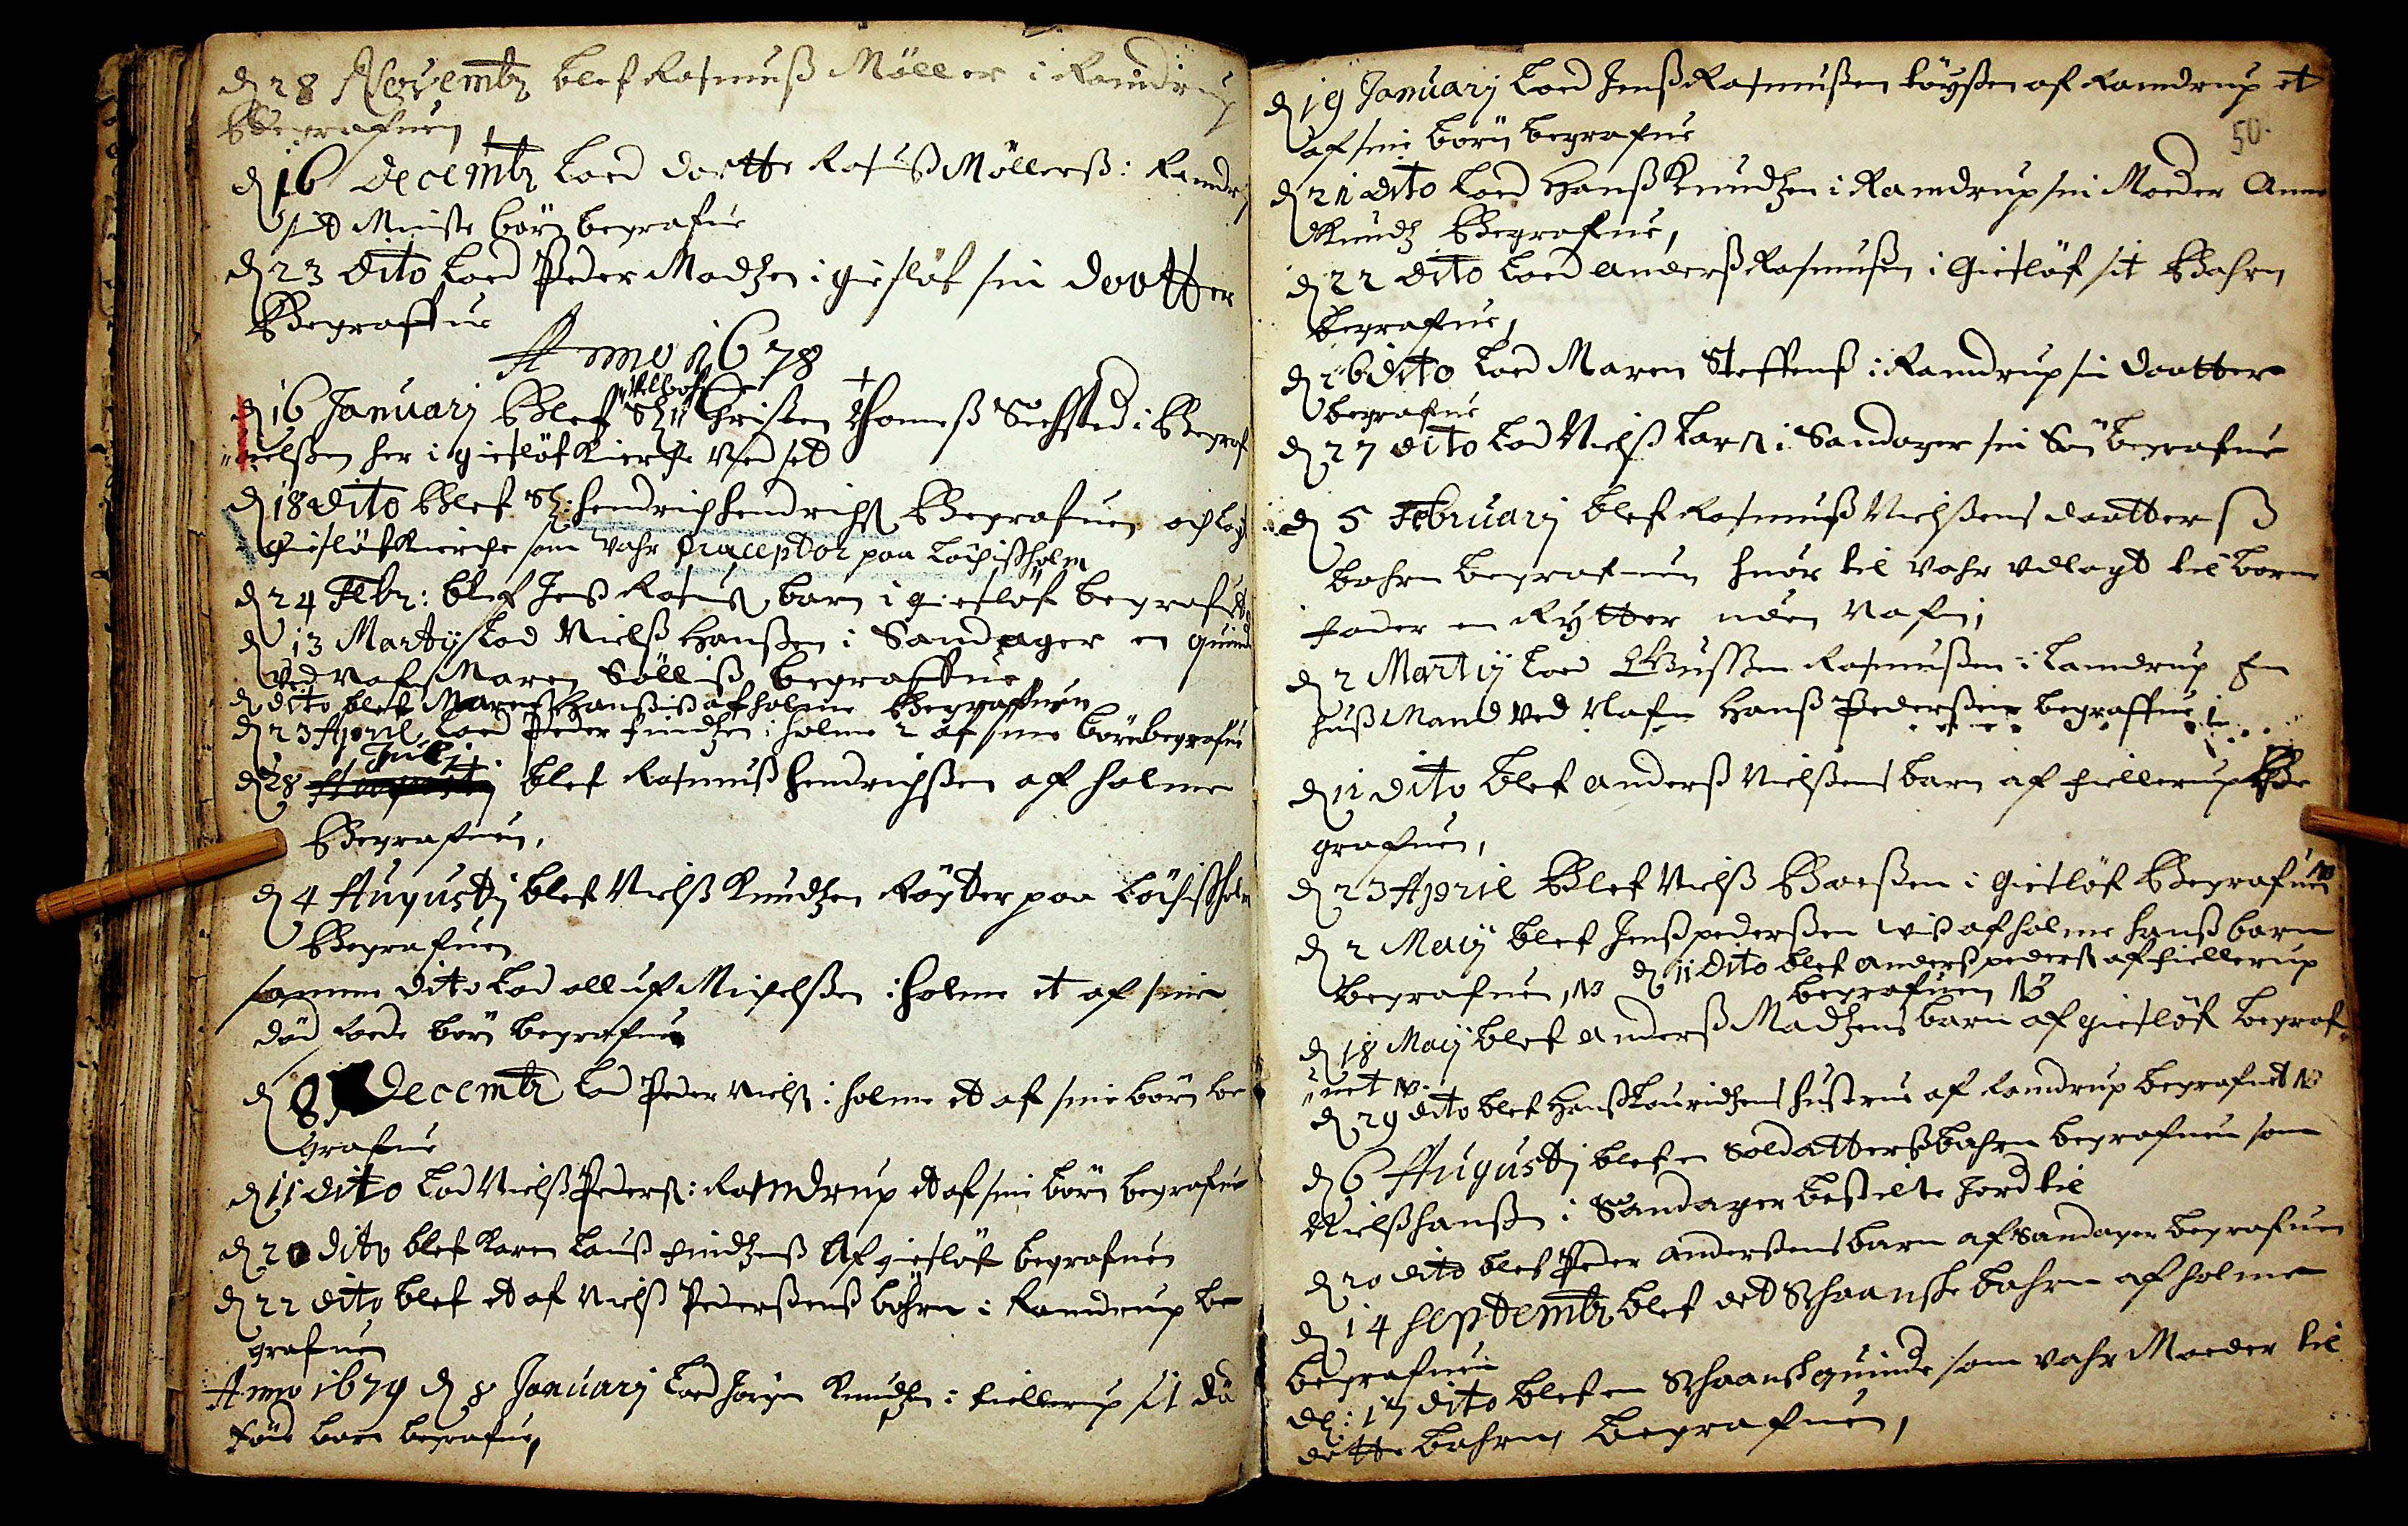d 28 Novembr blef Rasmuss Møller i Ramdrup
Begrafuen
d 16 Decembr loed dortte Rasmuss Møllers i Ramdrup
sit Minste børn begrafue
d 23 Dito Loed Peder Madtzen i giesløf sin daattter
Begraffue
Anno 1678
Velbohrne
d 16 Januarij Bleff ,, Christen Tomess Seehsted i Begraf
= uelssen her i gietsøf Kierche ned set
d 18 dito Bleff Sl: Hendrich Hendrichs Begrafuen och lagt
i Giesløf kierche som vahr Praceptor paa Løchisholm
d 24 Febr: blef Jens Rasmuss barn i giesløf begrafuet
d 13 Martij lod Nielss Hansen i Sandvger en quinde
ved nafn Maren Sølliss begraffue
d dito blef Maren Hanssis af holme begraffuen
d 23 April lod Peder Findtzen i holme 2 af sine børn begrafvue
Julj
d 8 Augustij blef Rasmuss Hendrichsen af holme
Begraffuen.
d 4 Augustij blef Nielss Knudtzen Røgter paa Løchisholm
Begrafuen
samme dito Lod olluff Michelssen i holme et af sine
død foede børn begraffue
d 8 Decembr lod Peder Nielss i holme et af sene born be
grafue
d 11 dito Lod Niels Peders i Ramdrup et af sine børn begrafue
d 20 dito blef Karen Lauss Fidztenss Af giesløf begrafuen
d 22 dito blef et af Niels Pederssens  bøhrn i Ramdrup be
grafnen
Anno 1679 d 8 Januarij lod Jørgen Knudtzen i Fiellerup sit død
føde barn begrafuen
50.
d 19 Januarij loed Jenss Rasmussen tøysten af Ramdrup et
af sine børn begrafue
d21 Dito loed Hanss Knudtzen i Ramdrup sin Moeder Anne
Knudtz begraffue,
d 22 Dito loed Anderss Rasmussen i Gietløf sit Bahrn
begraffue,
d 26 dito loed Maren Steffenss i Ramdrup sin daatter
begraffuet
d 27 dito lod Nielss Larss i Sandager sin Søn begrafue
d 5 Februarij blef Rasmuss Nielssens daatterss
bahrn begrafuen huor til vahr vdlagt til barne
fader en Rytter uden Nafn;
d 2 Martij loed Gustssen Rasmussen i Lamdrup en
Huss Mand ved Nafn Hanss Pederssen begraffue;
d 11 dito blef Anderss Nielssens barn af Fiellerup Be
grafuen,
d 23 Apriil Blef Nielss Boessen i Giesløf Begrafuen
d 2 Maij blef Jenss pederssen ##is af holme hHanss barn
begrafuen. NB d 11 Dito blef Anderss peders af Fiellerup
begrafuen NB
d 18 Maij blef Anderss Madtzens barn af giesløf begraf¬
= uet NB.
d 29 dito blef Hanss Louridtzens hustrue af Ramdrup begrafuet NB
d 6 Augustij blef en Soldatterss bahrn begrafuen som
Nielss Hansen i Sandager bestielte Jord til
d 20 dito blef Peder Andersens barn af Sandagen begrafuen
d. 14 Septembr blef det Schaanske bahrn af holme
begrafven
de: 17. dito bleff en Schaansk quinde som vafr Morder til
dette bahrn begrafuen
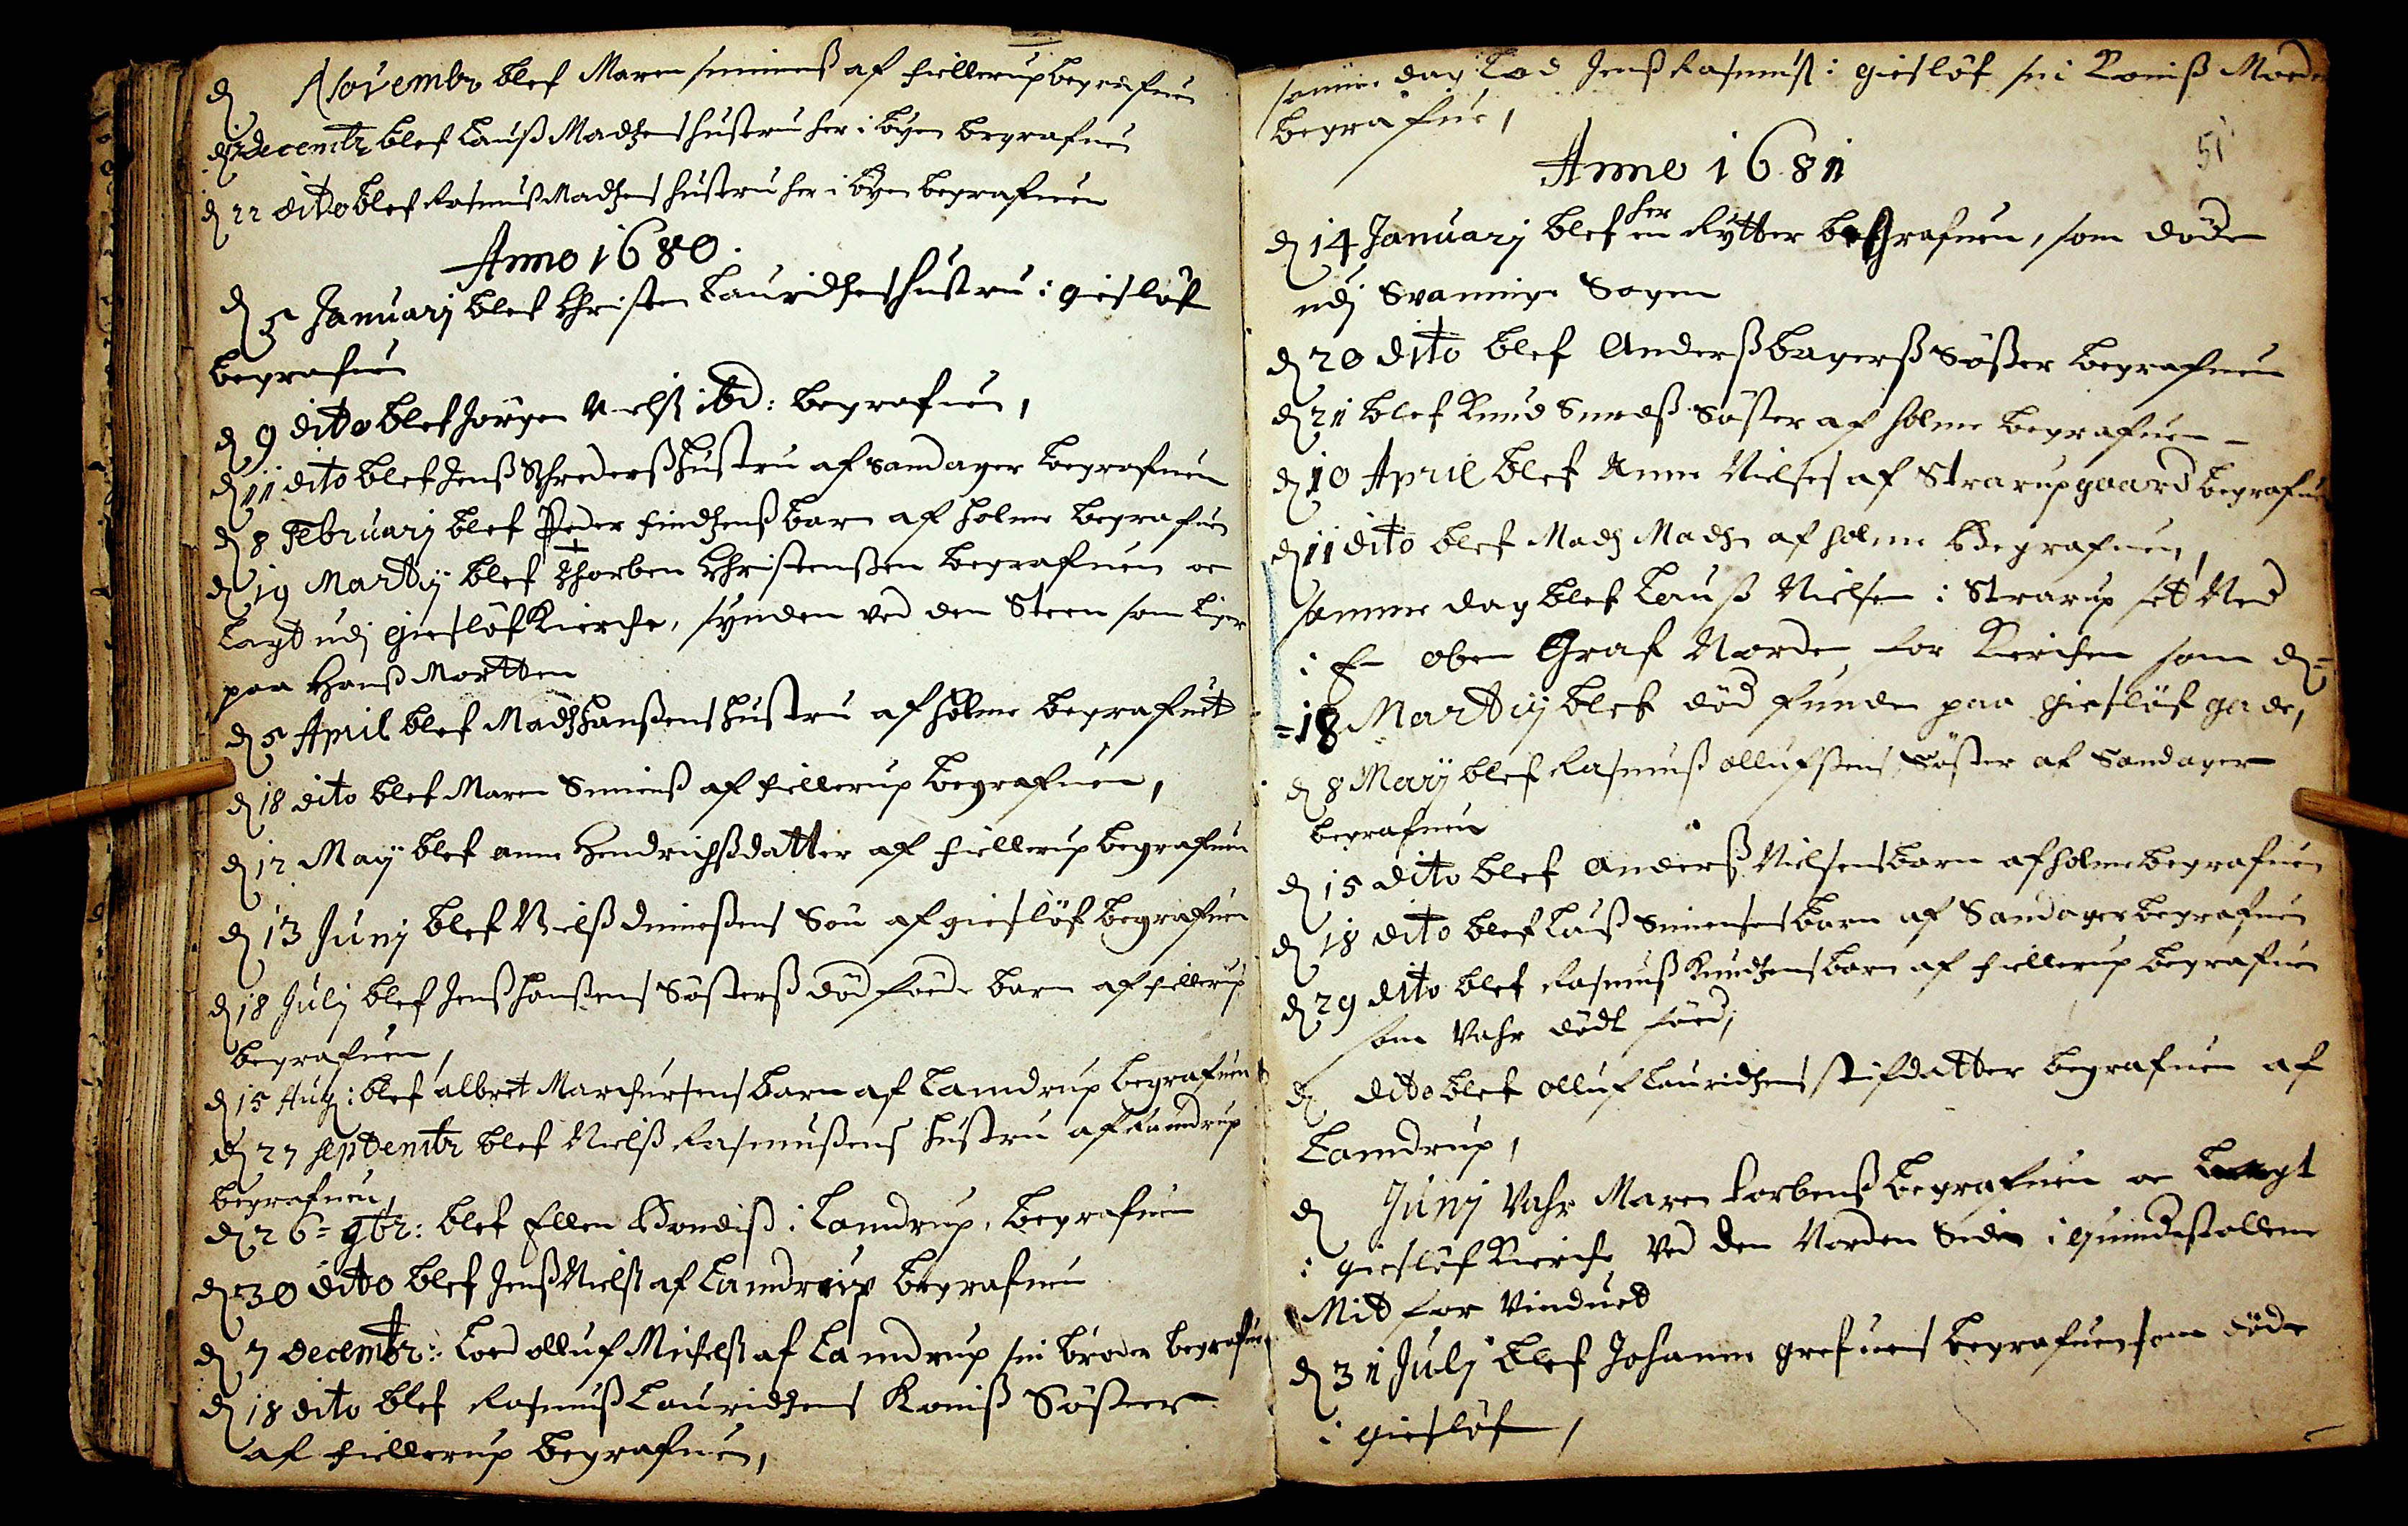d Novembr blef Maren simmenss af Fiellerup begrafuen
d 17 decembr blef Lauss Madtzens hustens her i byen begrafuen
d 22 dito blef Rasmuss Madtzens hustru her i byen begraffuen
Anno 1680
d 5 Januarij blef Christen Lauridtzens hustru i giesløf
begrafuen
d 9 dito blef Jørgen Nielss ibd. begrafuen,
d 11 dito blef Jens Schrederss Hustru af sandager begraffuen
d 8 Februarij blef Peder Findtzens barn af holme begraffuen
D 19 Martij blef Thorben Christenssen begrafuen oc
lagt udj Gisløf kierche synden ved den Streen som lig##
paa Hanss Mortten
d 5 April bleff Mads Hanssens Hustru af holme begraffuet
d 18 dito blef Maren Siminss af Fiellerup begrafuen,
d 12 Maij blef Anne Hendrichssdatter af Fiellerup begraffuen
d 13 Junij blef Nielss Dinnessens Søn af giesløf begraffuen
d 18 Julij blef Jens Hanssens Søsterss død føde barn aff Fiellerup
begrafuen
d 15 Aug: blef Albret Marchursens barn af Lamdrup begrafven
d 27 Septembr blef Nielss Rasmussens Hustru af Ramdrup
begrafuen
d 26 = 9br: blef Ellen Bondis i Lamdrup, begraffuen
d 30 dito blef Jens Nielss af Lamdrerip begraffen
d 7 Decembr: loed Olluf Michelss af Lamdrup sin Broder begrafue
d 18 dito blef Rasmuss Lauridtzens Konis Søster
af Fiellerup begrafen,
51
samme dag lod Jenss Rasmuss i Giesløf sin i Koniss
begrafue,
Anno 1681
her
d 14 Januarij blef en Rytter begraffuen, som døde
udj Svanninge Sogen
d 20 dito blef Anderss Bagerss Søster begraffuen
d 21 blef Knud Smeds Søster af holme begraffuen
d 10 April blef Anne Nielses af Strarpgaard begrafuen
d  11dito blef Mads Mads af holme Begrafuen
Samme dag blef Lauss Nielsen i Strarup set ved
i en oben Graf Norden for Kirchen som d=
= 18 Martij blef død funden paa giesløf gade,
d 8 Marij blef Rasmus Ollufssens Søster af Sandager
begrafuen
d 15 dito blef Anderss Nielsen barn af holm begrafuen
d 18 dito blef Lauss Simensens barn af Sandager begrafuen
d 29 dito blef Rasmuss Kudtzens barn af Fiellerup begraffuen
som vahr dødt føed;
d dito blef Olluf Lauridtzen stifdatter begrafuen af
Lamdrup,
d Junij Vahr Maren torbenss begrafuen oc Lagt
i Giesløf Kierche ved den Norden Side i quindestollene
Mit for Vinduet
d 31 Julij blef Johanne Grefuers begrafuen som døde
i Giesløf